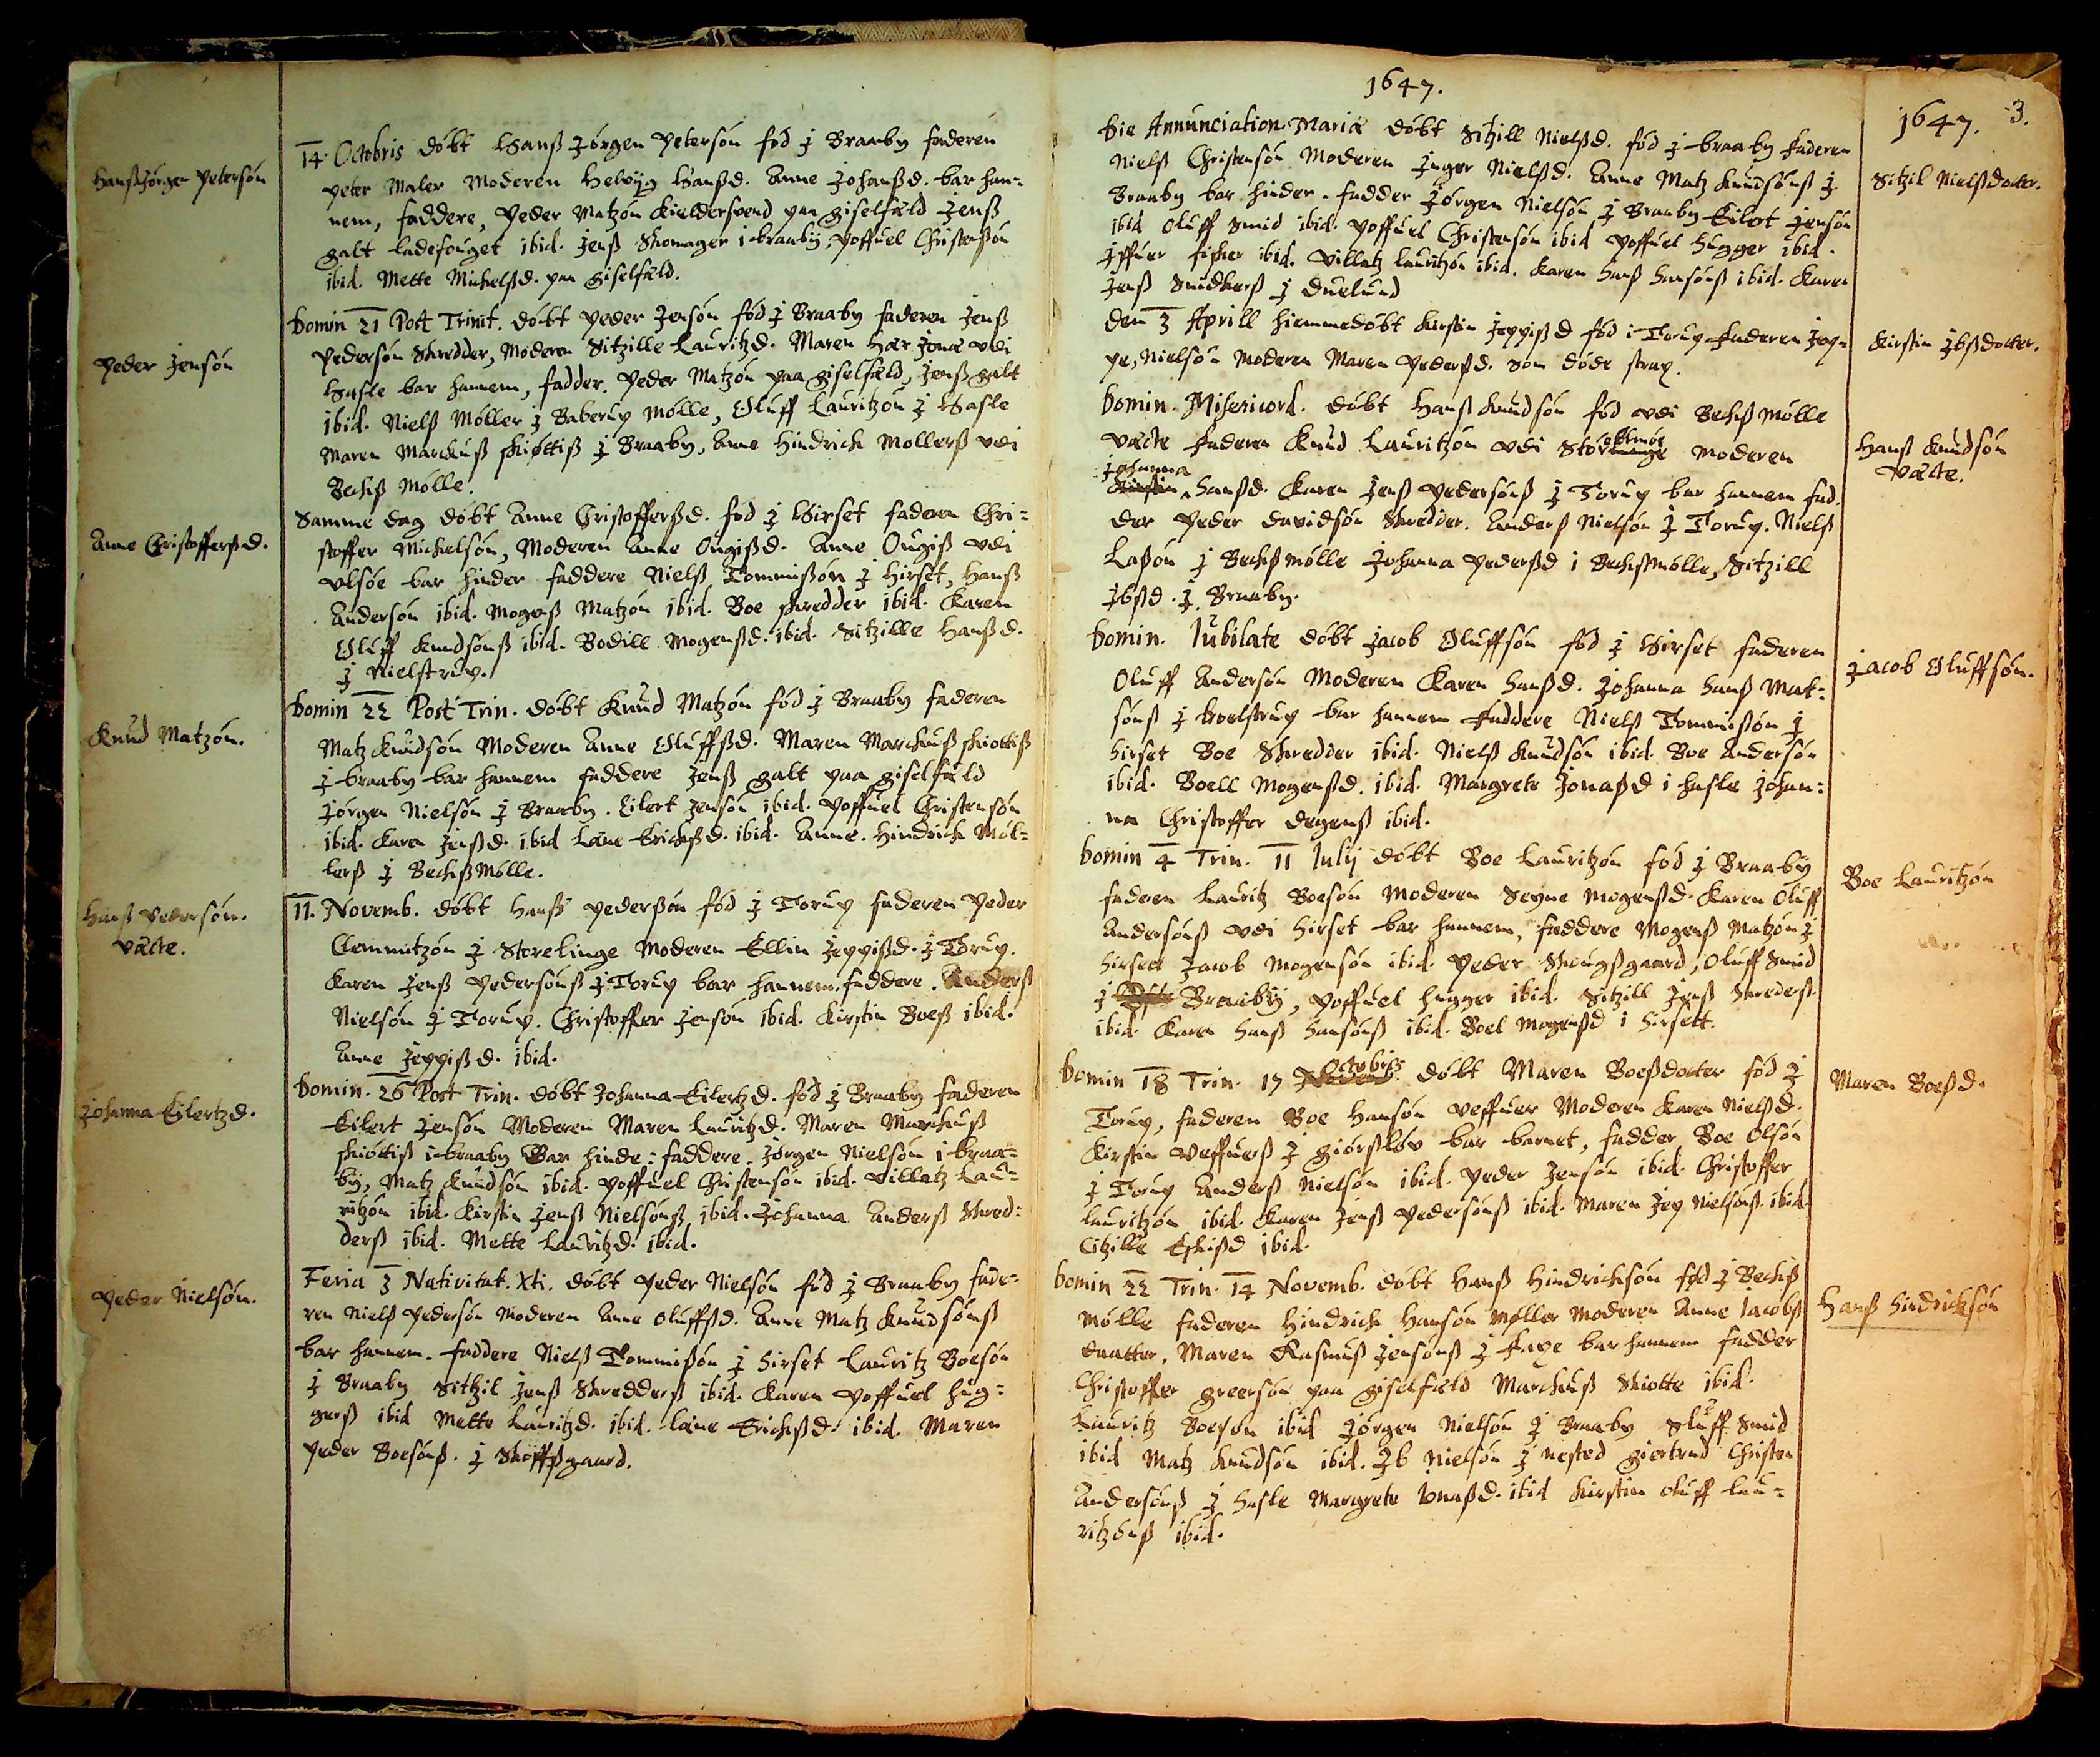Hans Jørgen Pedersøn 
14 . Octobris døbt Hans Jørgen Petersøn fød I Braaby Faderen
Peder Maler Moderen Helvij Hansd. Anne Johansd. bar han¬
nem, Faddere: Peder Matzøn Kieldersvend paa Giselfæld, Jens 
Galt Ladefoged ibid. Jens Skomager i Braaby, Poffuel Christensøn
ibid. Mette Micheld. paa Giselfæld. 
Peder Jensøn 
Domin 21 Post. Trinit. døbt Peder Jensøn fød I Braaby. Faderen Jens 
Pedersøn Skrædder, Moderen Sitzille Lauritzd. Maren Hær Jonæ## udi 
Hasle bar hannem, Fadder.Peder Matzøn paa Giselfæld, Jens Galt
ibid, Niels Møller J Baberup Mølle, Oluff Lauritzøn I Hasle
Maren Marckus Skiøttis I Braaby, Anne Hindrich Møllers udi 
Becks Mølle. 
Anne Christoffersd. 
Samme dag døbt Anne Christoffersd. fød I Hirset Fader Chri¬
stoffer Michelsøn, Moderen Anne Ougisd. Anne Ougis udi 
Ulsøe bar hinder. Faddere: Niels Tommissøn I Hirset, Hans 
Andersøn ibid. Mogens Matzøn ibid. Boe Skredder ibid. Karen 
Oluff Knudsøns ibid. Bodill Mogensd. ibid. Sitzille Hansd.
I Nielstrup. 
Knud Matzøn 
Domin 22 Post. Trin. døbt Knud Matzøn fød J Braaby Faderen 
Matz Knudsøn Moderen Anne Oluffssd. Maren Marchus Skiøttis 
I Braaby bar hannem Faddere: Jens Galt paa Giselfæld
Jørgen Nielsen I Braaby. Eilert Jensøn ibid, Poffuel Christensøn 
ibid. Karen Jensd. ibid, Læne Erichsd. ibid. Anne Hindrich Møl¬
lers I Bechs Mølle. 
Hans Pedersøn 
uæcte 
11. Novemb. døbt Hans Pedersøn fød I Torup. Faderen Peder
Clemmitzøn I Skrelinge. Moderen Ellin Jeppisd.  I Torup.
Karen Jens Pedersøns I Torup bar hannem. Faddere. Anders
Nielsøn I Torup. Christoffer Jensøn ibid. Kirstin Boes ibid. 
Anne Jeppisd. ibid. 
Johanne Eilertzd.
Domin 26 Post Trin. døbt Johanne Eilertzd. fød I Braaby. Faderen 
Eilertz Jensøn Moderen Maren Lauritzd. Maren Marchus 
Skiøttis i Braaby bar hinde. Faddere Jørgen Nielsøn i Braa¬
by, Matz Knudsøn ibid. Poffuel Christensøn, ibid. Villatz Lau¬
ritzøn, ibid. Kirstin Jens Nielsøn, ibid. Johanna Anders Skræd¬
ders, ibid. Mette Lauritzd. ibid. 
Peder Nielsøn 
Feria 3 Nativitat. Xti. døbt Peder Nielsøn fød I Braaby. Fade¬
ren Niels Pedersøn. Moderen Ane Oluffsd. Anne Matz Knudsøns
bar hannem. Faddere: Niels Tommisøn I Hirset. Lauritz Boesøn 
I Braaby. Sitzil Jens Skrædders ibid. Karen Poffuel hug¬
gers ibid. Mette Lauritzd. ibid. Læne Erichsd. ibid. Maren 
Peder Boesøns I Skoffsgaard. 
1647.
Die Annunciatio Mariæ døbt Sitzel Nielsd. fød I Braaby Faderen 
Niels Christensøn. Moderen Inger Nielsd. Anne Matz Knudsis I
Braaby bar hinder. Fadder Jørgen Nielsøn I Braaby. Eilert Jensøn
ibid. Oluff Smid ibid. Poffuel Christensøn ibid. Poffuel Hugger ibid.
Iffur Fisker ibid. Villatz Lauritzøn, ibid. Karen Hans Hansøns ibid. Karen 
Jens Snidkers I Duelund
3
1647.
Sitzel Nielsdatter 
Den 3. Aprill Hiemmedøbt Kirstin Jeppisd. fød i Torup. Faderen Jep¬
pe Nielsøn Moderen Maren Pedersd. som døde strax. 
Kirstin Jepsdatter 
Domin. Misericord. døbt Hans Knudsøn fød udi Becks Mølle 
##
uæcte. Faderen Knud Lauritzøn udi St##. Moderen 
Johanna
## Hansd. Karen Jens Pedersøns I Torup bar hannem. Fad¬
der Peder Davidsøn Skrædder. Anders Nielsøn I Torup. Niels 
Lassøn I Becks Mølle. Johanna Pedersd. i Becksmølle. Sitzill
Ibsdatter I Braaby.
Hans Knudsøn
Uæcte
Domin. Jubilate døbt Jacob Oluffsøn fød I Hirset. Faderen 
Oluff Andersøn. Moderen Karen Hansd. Johanna Hans Mat¬
søns I Troelsrup bar hannem. Faddere Niels Tommisøn I 
Hirset. Boe Skrædder ibid. Niels Knudsøn ibid. Boe Andersøn
ibid. Boell Mogensd. ibid. Margrete Jonasd. i Hasle. Johan¬
na Christoffer Degens, ibid. 
Jacob Oluffsøn 
Domin 4 Trin. 11 July døbt Boe Lauritzøn fød I Braaby
Faderen Lauritz Boesøn. Moderen Seigne Mogensd. Karen Oluff
Andersøns udi Hirset bar hannem. Faddere Mogens Matzøn I
Hirset. Jacob Mogensøn ibid. Peder Skougsgaard Oluff Smid
I ## Braaby, Poffuel hugger ibid. Sitzill Jens Skreders 
ibid. Karen Hans Hansøns ibid. Boel Mogensd. i Hirsett.
Boe Lauritzøn 
Octobris
Domin 18 Trin. 17 Novem døbt Maren Boesdatter fød I 
Torup, Faderen Boe Hansøn Veffuer Moderen Karen Nielsd. 
Kirstin Veffuers I Giørsløv bar barnet, Fadder Boe Olsøn
I Torup. Anders Nielsøn ibid. Peder Jensøn ibid. Christoffer 
Lauritzøn ibid. Karen Jens Pedersøns ibid. Maren Jep Nielsøns ibid.
Citzille Eskisd. ibid. 
Maren Boesdatter 
Domin 22 Trin. 14. Novemb. døbt Hans Hindricksøn fød I Becks
Mølle. Faderen Hindrick Hansøn Møller. Moderen Anne Jacobs¬
daatter. Maren Rasmus Jensøns I Faxe bar hannem. Fadder
Christoffer Greersøn paa Giselfæld Marckus Skiotte ibid.
Lauritz Boesøn ibid Jørgen Nielsøn I Braaby. Oluff Smid
ibid Matz Knudsøn ibid. Ib Nielsøn i Nested Giertrud Christen 
Andersøns i Hasle. Margrete Jonasd. ibid Kirstin Oluff Lau¬
ritzsens ibid. 
Hans Hindricksøn